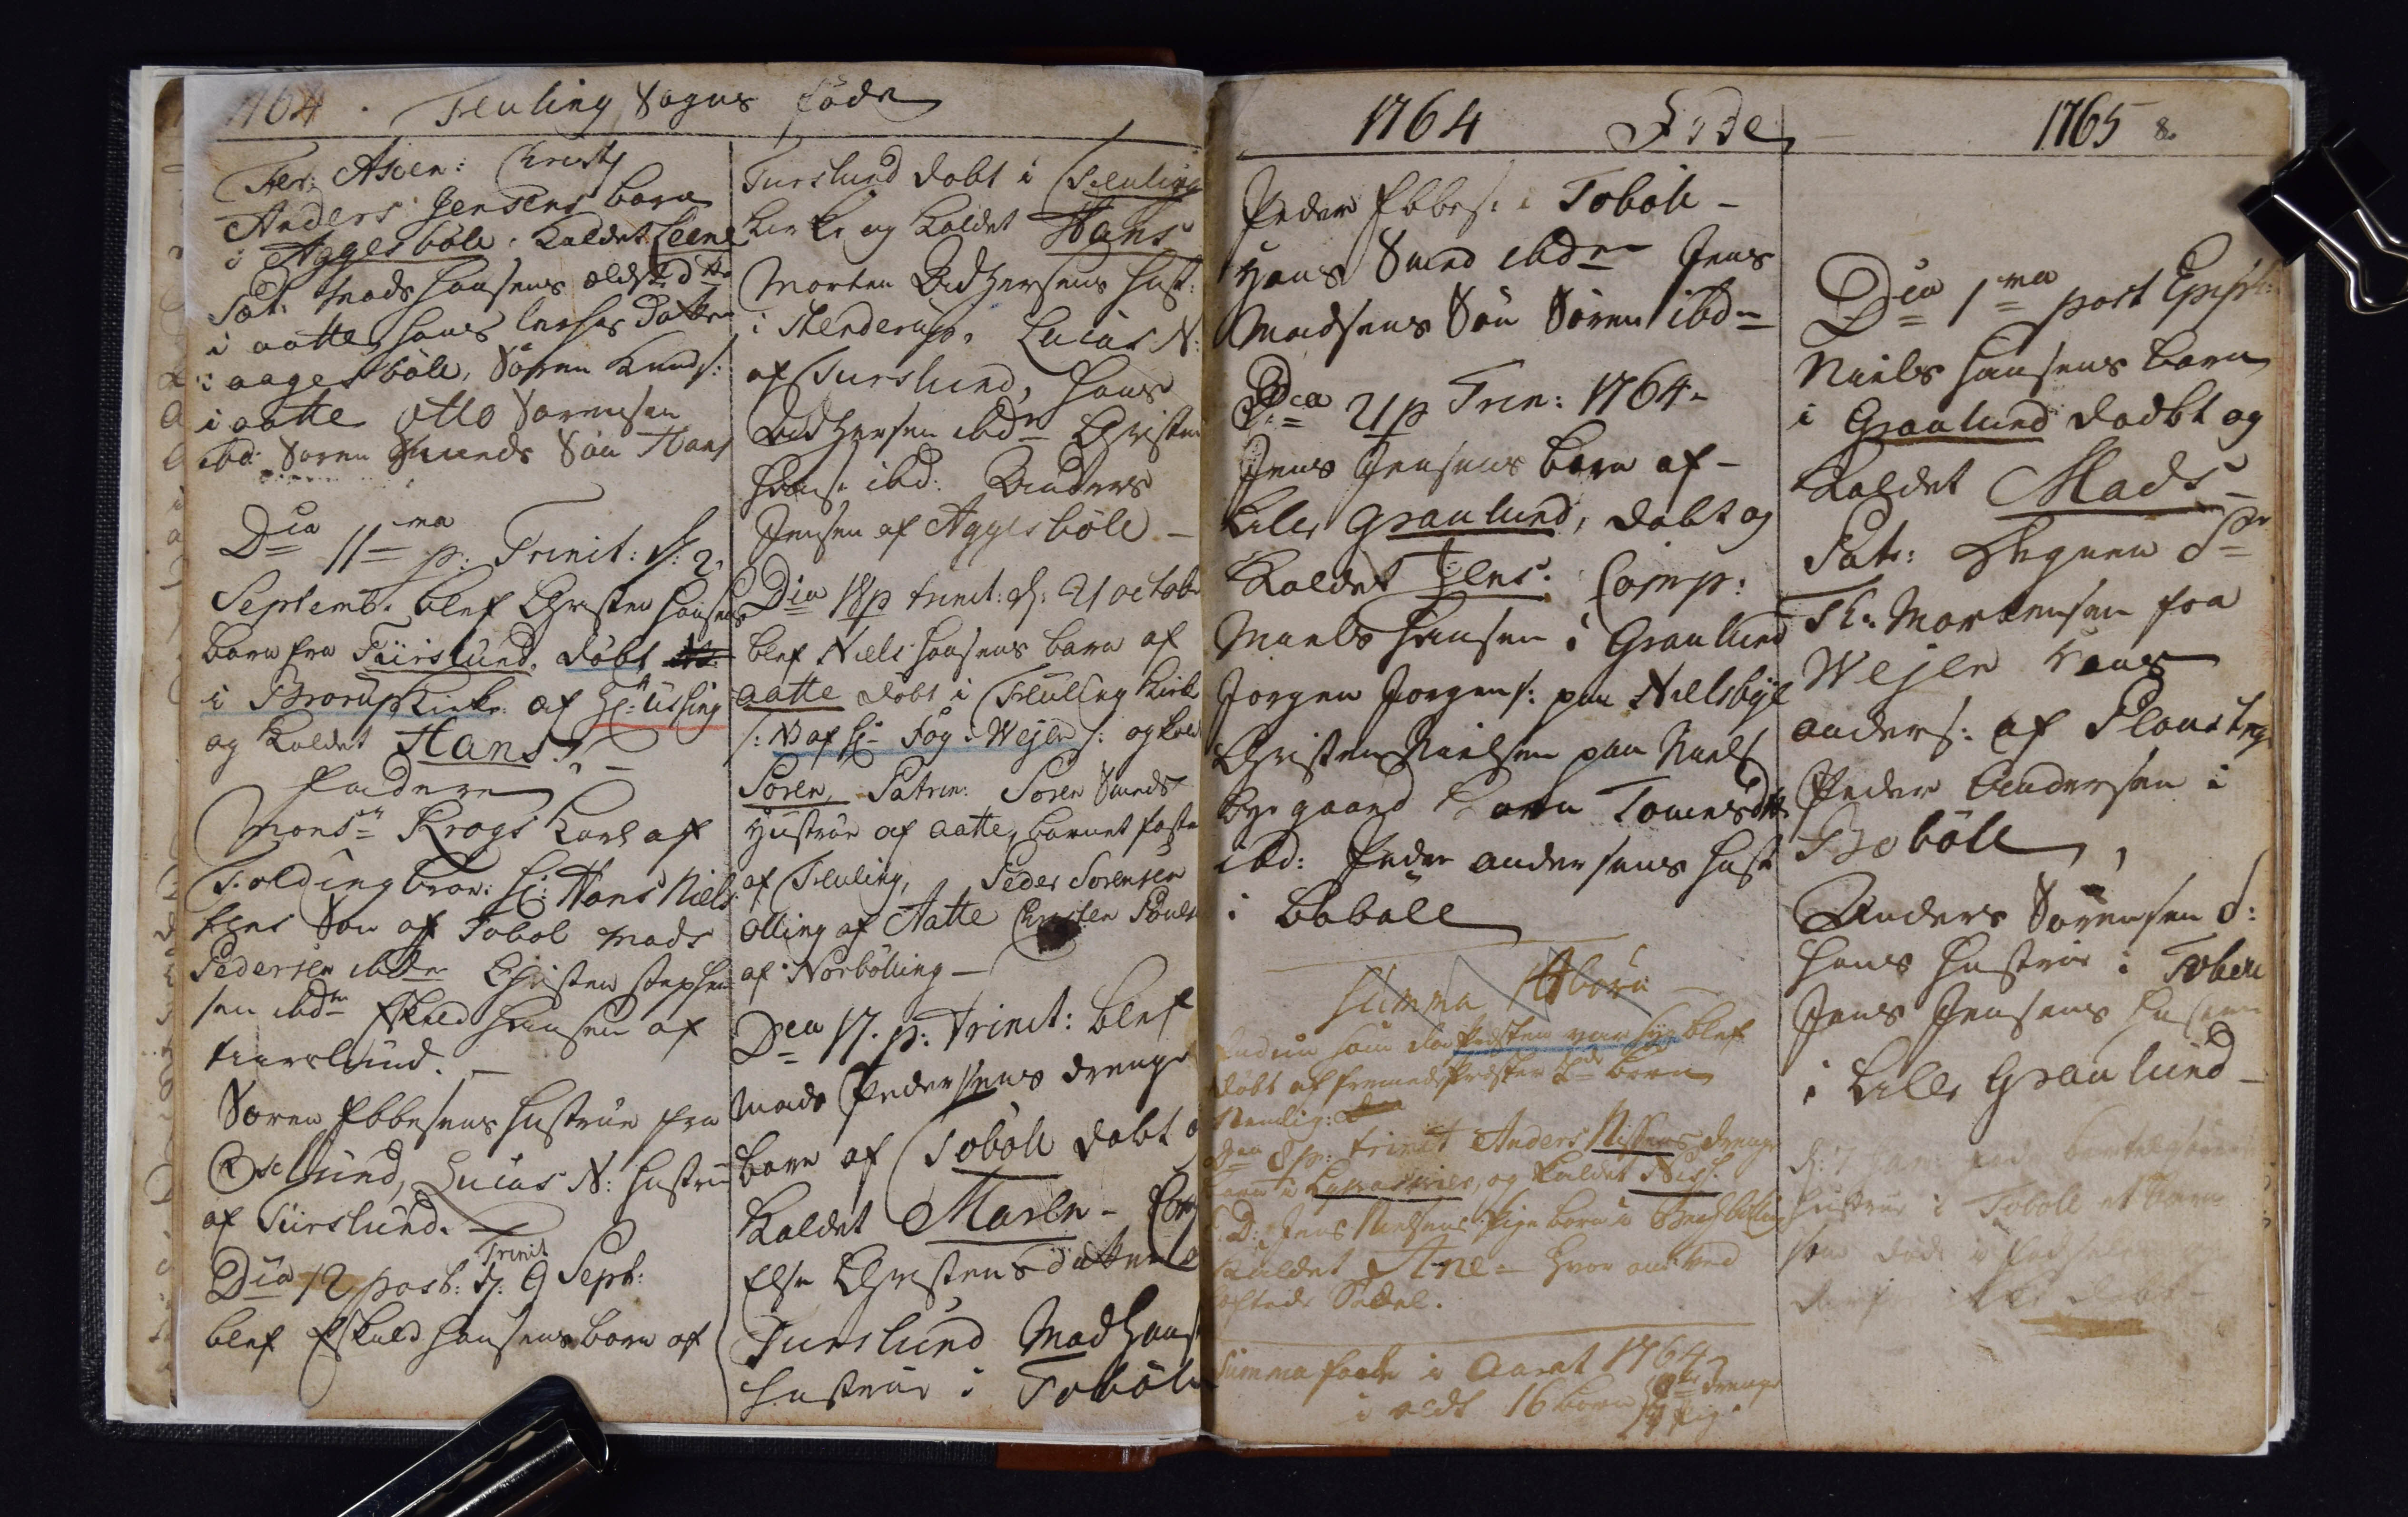1764
Feuling Sogns føde
Fer: Ascen: Christj
Anders Jensens barn
i Aggesbøll kaldet Leene
sat: Mads Hansens ældste Dtr
i aatte Hans Lerhes Datter
i aagesbøll, Søren Knuds:
i aatte Otto Sørensen
ibd: Søren Smeds Søn Hans
Dca 11ma p. Trinit: d: 2
Septemb. blef Christen Hansens
barn fra Tiirslund. døbt ##
i Brorup Kirke af Hl: Ussing
og kaldet Hans.
Faddere
Mons## Krogs Karl af
Folding Kroe: Hl: Hans Niels
sens Søn af Tobol Mads
Pedersen ibdm Christen Stephen
sen ibdm Eskild Hansen af
tiirslund.
Søren Ebbesens Hustrue fra
O##lund, Lucas N: Hustru
af Tiirslund.
Trinit
Dca 12 post d: 9 Sept:
blef Eskild Hansens barn af
Tiirslunf døbt i Feuling
Kirke og Kaldet Hans
Morten Adtzersens Hust:
i Stenderup, Lucas N:
af Tiirslund, Hans
Adtzersen ibdn Christen
Hans ibd: Anders
Jensen af Aggesbøll -
Dca 18 p Trinit: d: 21 octobr
blef Niels Hansens barn af
aatte døbt i Feuling Kirk
/: NB af Hl: Fog i Wejen /: og kald
Søren, Patrin. Søren Smeds
Hustrue af Aatte, barnet Faste
af Feuling, Peder Sørensen
Olling af Aatte Christen Povls
af Norbølling -
Dca 17 p: Trinit: Blef
Mads Pedersens Drenge
Barn af Tobøll døbt o
kaldet Maren - Com##
Else Christensdatter
Tiirslund Madhans
Hustrue i Tobøll
1764
Føde
Peder Ebbesen i Tobøll
Hans Smed ibd## Jens
Madsens Søn Søren ibd##
Dca 21 p Trin: 1764.
Jens Jensens barn af
Lille Graulund, døbt og
kaldet Jens. Comp:
Mads Hansen i Graulund
Jørgen Jørgens paa Nielsbye
Christen Nielsen paa Niels
bye gaard Karen Tomesdtr
ibd: Peder Andersens Hustr
i Bobøll.
Summa 16 børn
Endnu som da## Præsten var syg blef
døbt af fremmed Præster 2de Born
Nemlig: ##
Dca 8 p: Trinit: Anders Nielsens Drenge
Barn i Lykeskier, og kaldet Niss.
D: Jens Nielsens Pige barn i Bechbølling
kaldet Ane:  hvor om ved
##æfeds Sædel
Summa faade i Aaret 1764
i alt 16 Born
9## drenge
7 piger
8.
1765
Dca 1ma post Epiph:
Niels Hansens barn
i Graulund døbt og
kaldet Mads
Pat. Degnen Sr
Th: Mortensen fra
Vejen Hans
Anders: af Ploustrup
Peder Anderssen i
Bobøll
Anders Sørensen ##:
Hans Hustrue i Tobell
Jens Jensens Hustru
i Lille Graulund -
d: 7 Jan: fod Bertel sørensens
Hustrue i Toboll et Barn
som døde i Fødselen og
derfor ikke døbt -
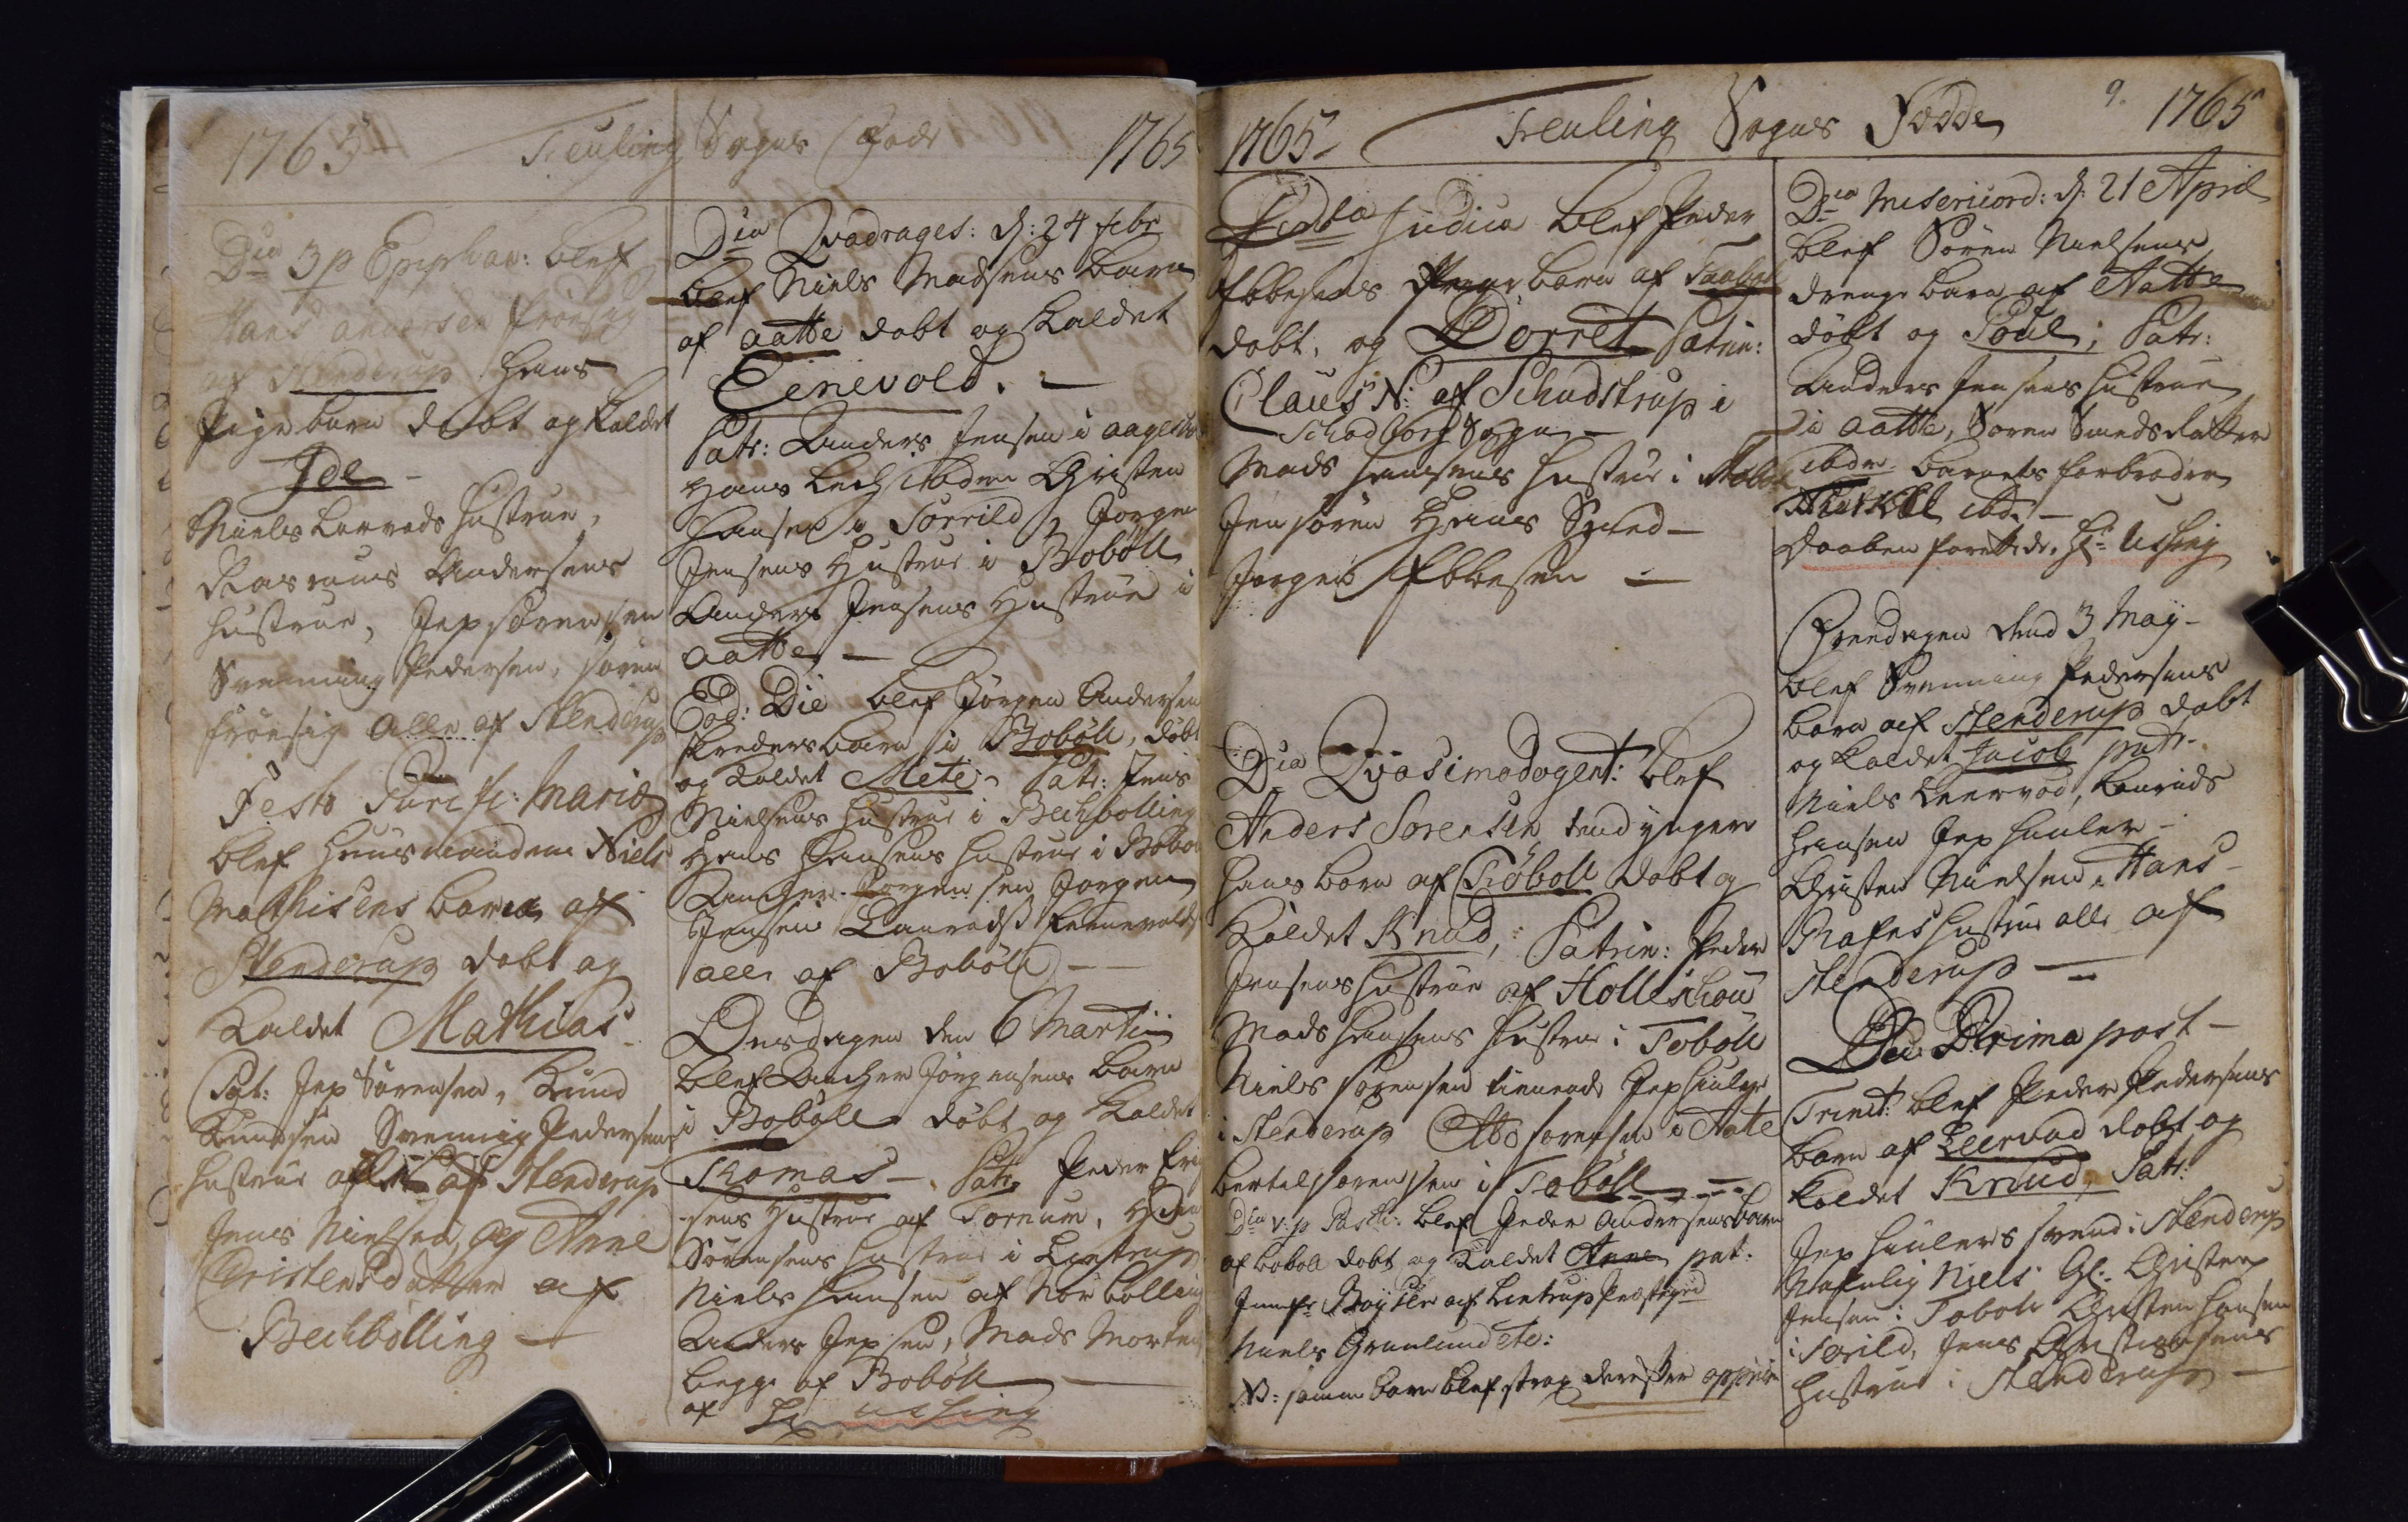1765
Feuling Sogns Fode
Dca 3 p Epiphan: blef
Hans Andersen Frøesig
af Stenderup Hans
Pigebarn døbt og kaldet
Ide
Niels Lervads Hustrue,
Rasmus Andersens
Hustrue, Jep Sørensøn
Svenning Pedersen; Søren
Frøsig, alle af Stenderup
Festo Purifc: Mariæ
blef Huusmanden Niels
Mathisens barn af
Stenderup døbt og
kaldet Mathias
Pat: Jep Sørensen, Knud
Kundsen, Svenning Pedersens
Hustrue alle af Stenderup
Jens Nielsen og Anne
Christensdatter af
Bechbølling
1765
Dca Qvadrages: d: 24 Febr
blef Niels Madsens Barn
af Aatte dobt og kaldet
Enevold.
Patr: Anders Jensen i Aagesbo##
Hans Lech ibdm Christen
Hansen i Sorrild, Jørgen
Jensens Hustrue i Bobøll
Anders Jensens Hustrue i
Aatte
Eod: Die blef Jørgen Andersen
Skreders Barn i Bobøll, døbt
og kaldet Mete Patr: Jens
Nielsens Hustrue i Becbølling
Hans Fransens Hustrue i Bobol
Anders Jorgensen Jorgen
Jensen Lauridss Eenuevolds
alle af Bobøll.
Onsdagen den 6 Martii
Blef Ancher Jørgensens Barn
i Bobøll. døbt og kaldet
Thomas - . Patr Peder Eri##
sens Hustrue af Toenum, Hans
Sørensens Hustrue i Lintrup.
Niels Hansen af Nørbølling
Anders Jepsen, Mads Mortens
begge af Bobøll
af Hl ussing
1765.
Feuling Sogns Fodde
## Judica blef Peder
Ebbesens Pige Barn af Taaboll
døbt, og Dorret Patrin:
Claus N: af Schodstrup i
Schodborg Sogn
Mads Hansens Hustru i Toboll
Jen Søren Hans Smed
Jørgen Ebbesen
Dca Qvasimodogent: blef
Anders Sørensen dend yngere
hans barn af Toboll døbt og
kaldet Knud, Patrin: Peder
Jensens Hustrue af Holleschou
Mads Hansens Hustru i Tobøll
Niels Sørensen tienende Jep Hiuler
i Stenderup Otto Sorensen i Aatte
Bertel Sørensen i Tobøl
Dca V: p Pasch: blef Peder Andersens barn
af Boboll dobt og kaldet Anne pat:
Jens Boysen af Lintrup Præstegrd
Niels Graulund etc:
NB: samme barn blef strax derefter opp##
9.
1765
Dca Misericord: d 21 April.
blef Søren Nielsens
Drengebarn af Aatte
døbt og Poul; Patr:
Anders Jensens Hustrue
i Aatte, Søren Smeds datter
ibdm Barnets farbroder
Thorkel ibid.-
Daaben forettede Hl: Ussing
Fredagen dend 3 Maij-
blef Svenning Pedersens
Barn af Stenderup dobt
og kaldet Jacob patr-
Niels Leervod Laurids
Hansen Jep Hiuler
Christen Nielssen, Hans
Rafns Hustrue alle af
Stenderup
Dca ##rima post -
Trinit: bleff Peder Pedersens
Barn af Leervad dobt og
kaldet Knud Patr::
Jep Hiulers Svend i Stenderup
Nafnlig Niels Gl: Christen
Jensen i Toboll Christen Hansen
i Sorild, Jens Christensens
Hustrue i Stenderup